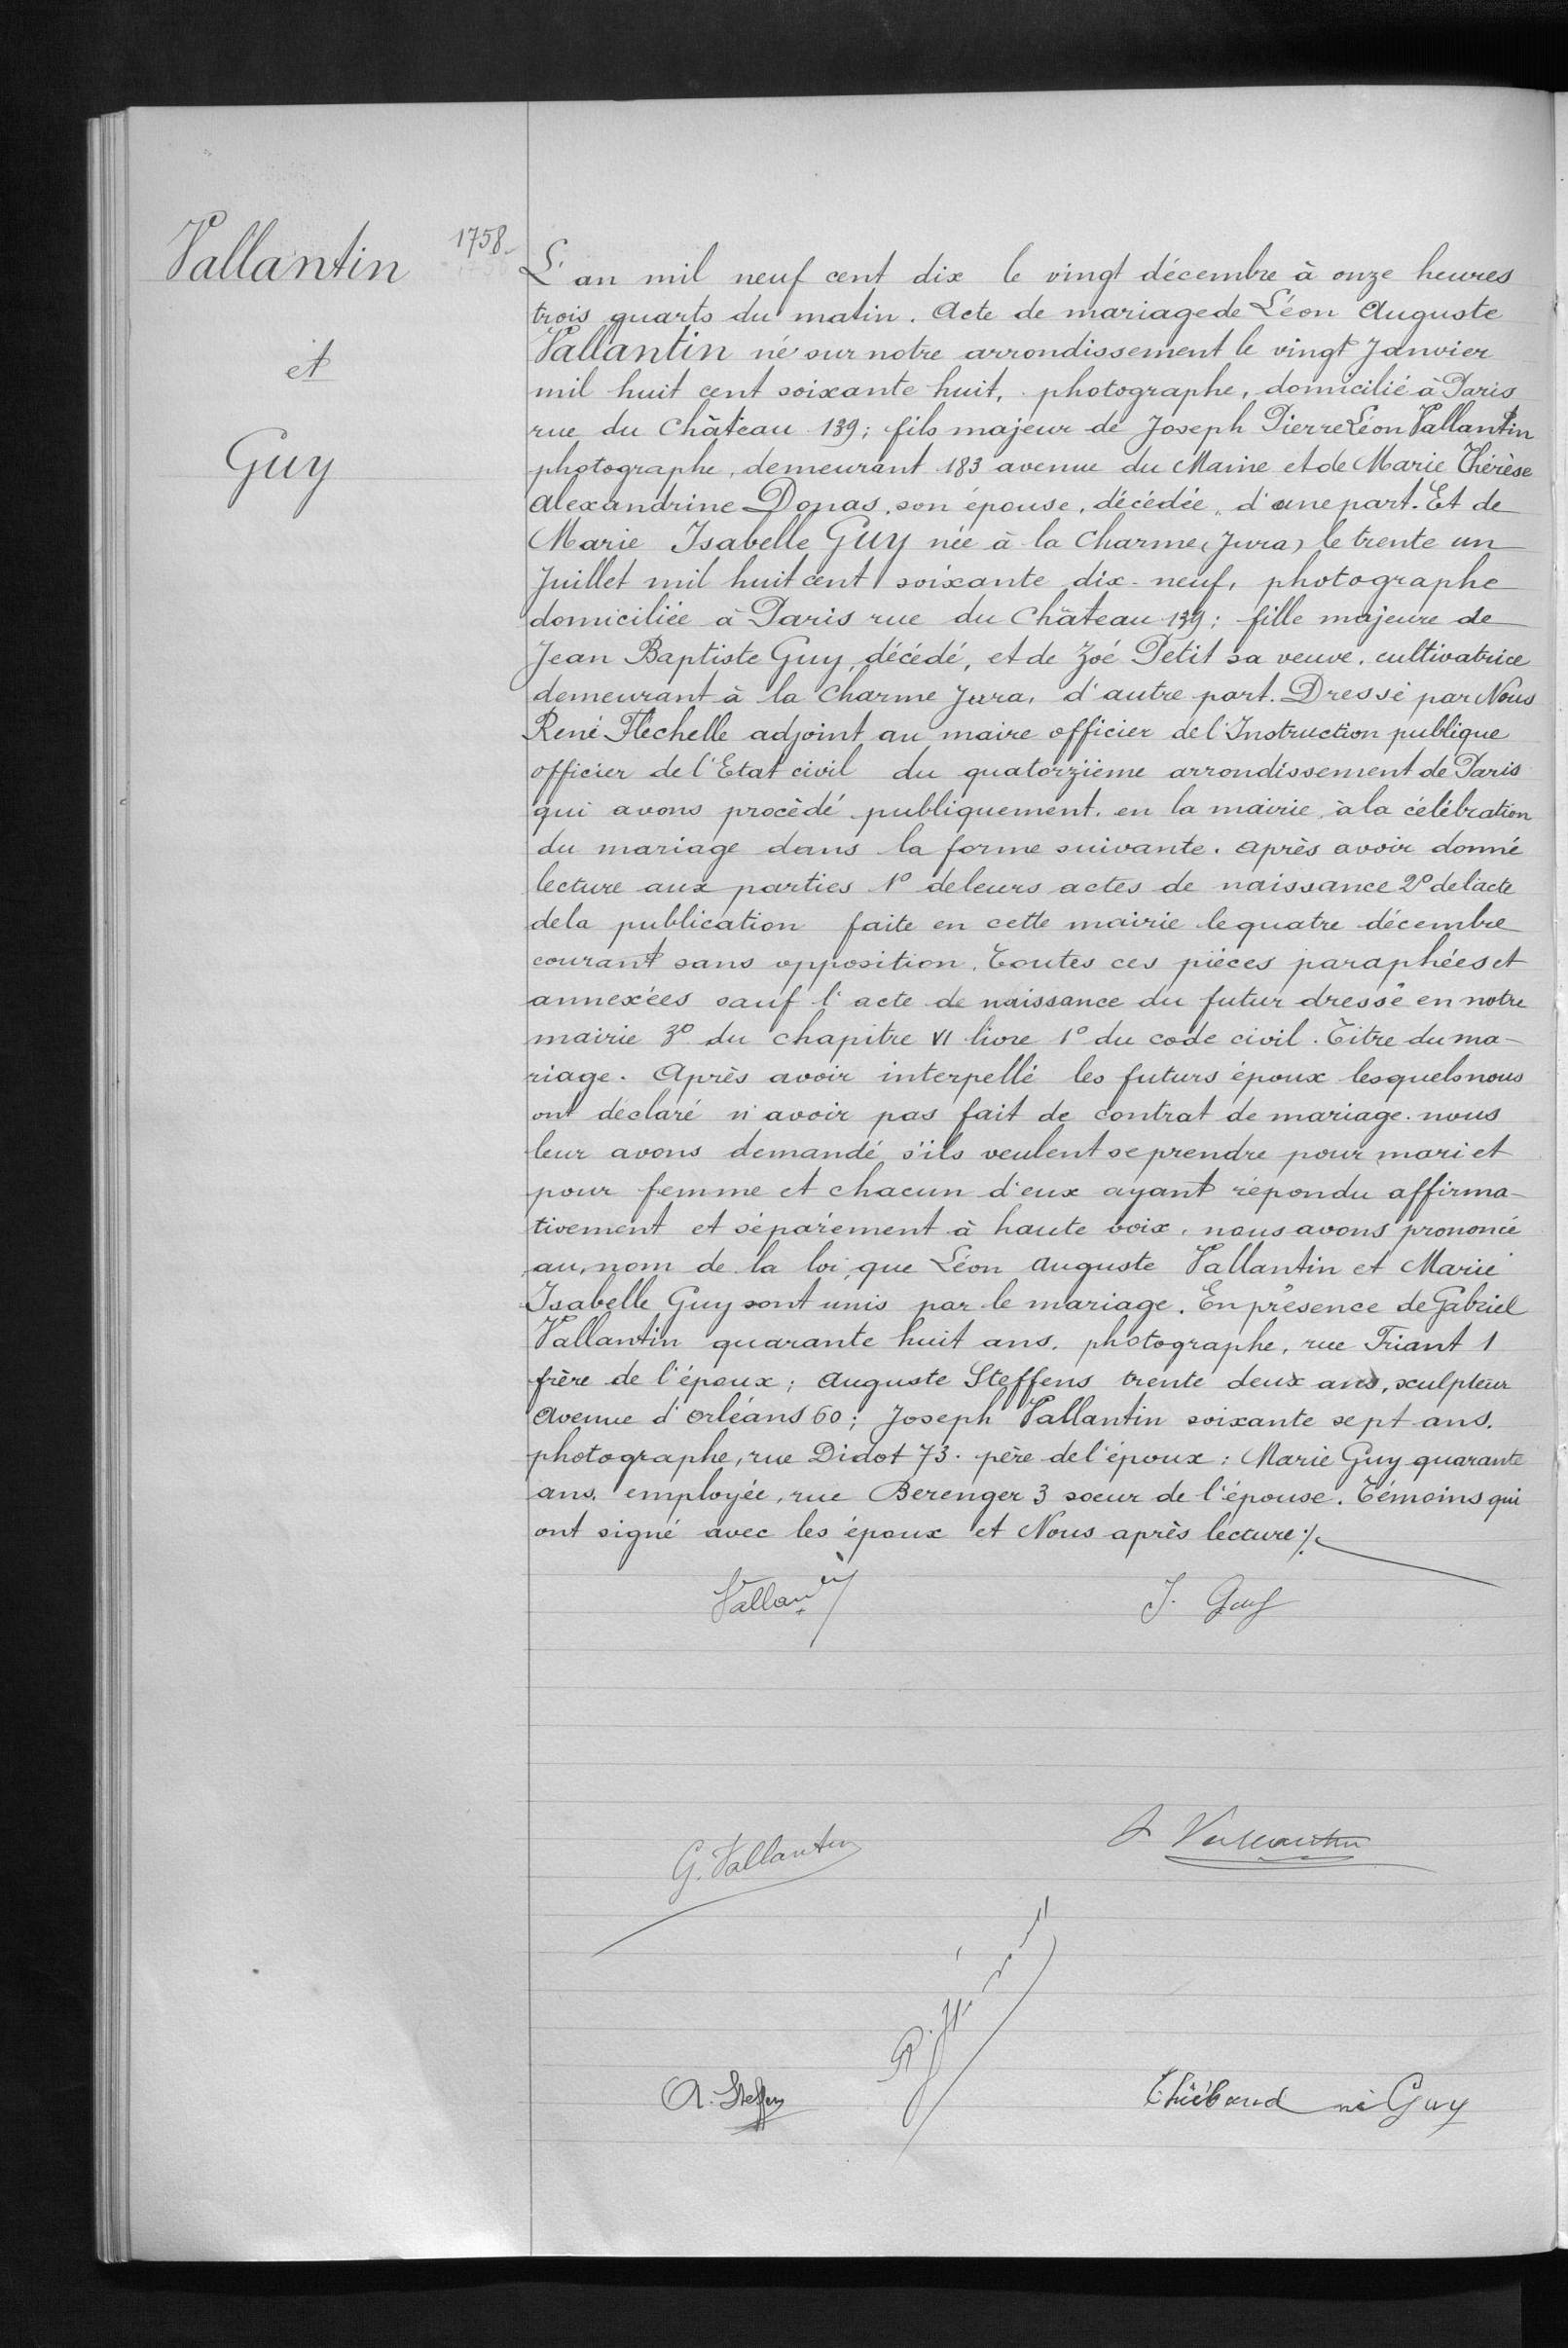1758
Vallantin
et
Guy
L'an mil neuf cent dix le vingt décembre à onze heures
trois quarts du matin. Acte de mariage de Léon Auguste
Vallantin né sur notre arrondissement le vingt Janvier
mil huit cent soixante huit, photographe, domicilié à Paris
rue du Château 139: fils majeur de Joseph Pierre Léon Vallantin
photographe, demeurant 183 avenue du Maire et de Marie Thérèse
Alexandrine Donas, son épouse, décédée, d'une part. Et de
Marie Isabelle Guy née à la Charme (Jura) le trente un
Juillet mil huit cent soixante dix-neuf, photographe
domiciliée à Paris rue du Château 139: fille majeure de
Jean Baptiste Guy, décédé, et de Zoé Petit sa veuve, cultivatrice
demeurant à la Charme Jura, d'autre part. Dressé par Nous
officier de l'Etat civil du quatorzième arrondissement de Paris
qui avons procédé publiquement en la mairie à la célébration
du mariage dans la forme suivante. après avoir donné
lecture aux parties 1° de leurs actes de naissance 2° de l'acte
de la publication faite en cette mairie le quatre décembre
courant sans opposition. Toutes ces pièces paraphées et
annexées sauf l'acte de naissance du futur dressé en notre
mairie 3° du chapitre VI livre 1° du code civil.; Titre du ma-
riage. Après avoir interpellé les futurs époux lesquels nous
ont déclaré n'avoir pas fait de contrat de mariage nous
leur avons demandé s'ils veulent se prendre pour mari et
pour femme et chacun d'eux ayant répondu affirma-
tivement et séparément à haute voix, nous avons prononcé
au nom de la loi que Léon Auguste Vallantin et Marie
Isabelle Guy sont unis par le mariage. En présence de Gabriel
Vallantin quarnte huit ans, photographe rue Friant 1
frère de l'époux: Auguste Steffens trente deux ans, sculpteur
Avenue d'Orléans 60; Joseph Vallantin soixante sept ans,
photographe, rue Didot 73, père de l'époux: Marie Guy quarante
ans, employée, rue Berenger 3 soeur de l'épouse, témoins qui
ont signé avec les époux et Nous après lecture./.
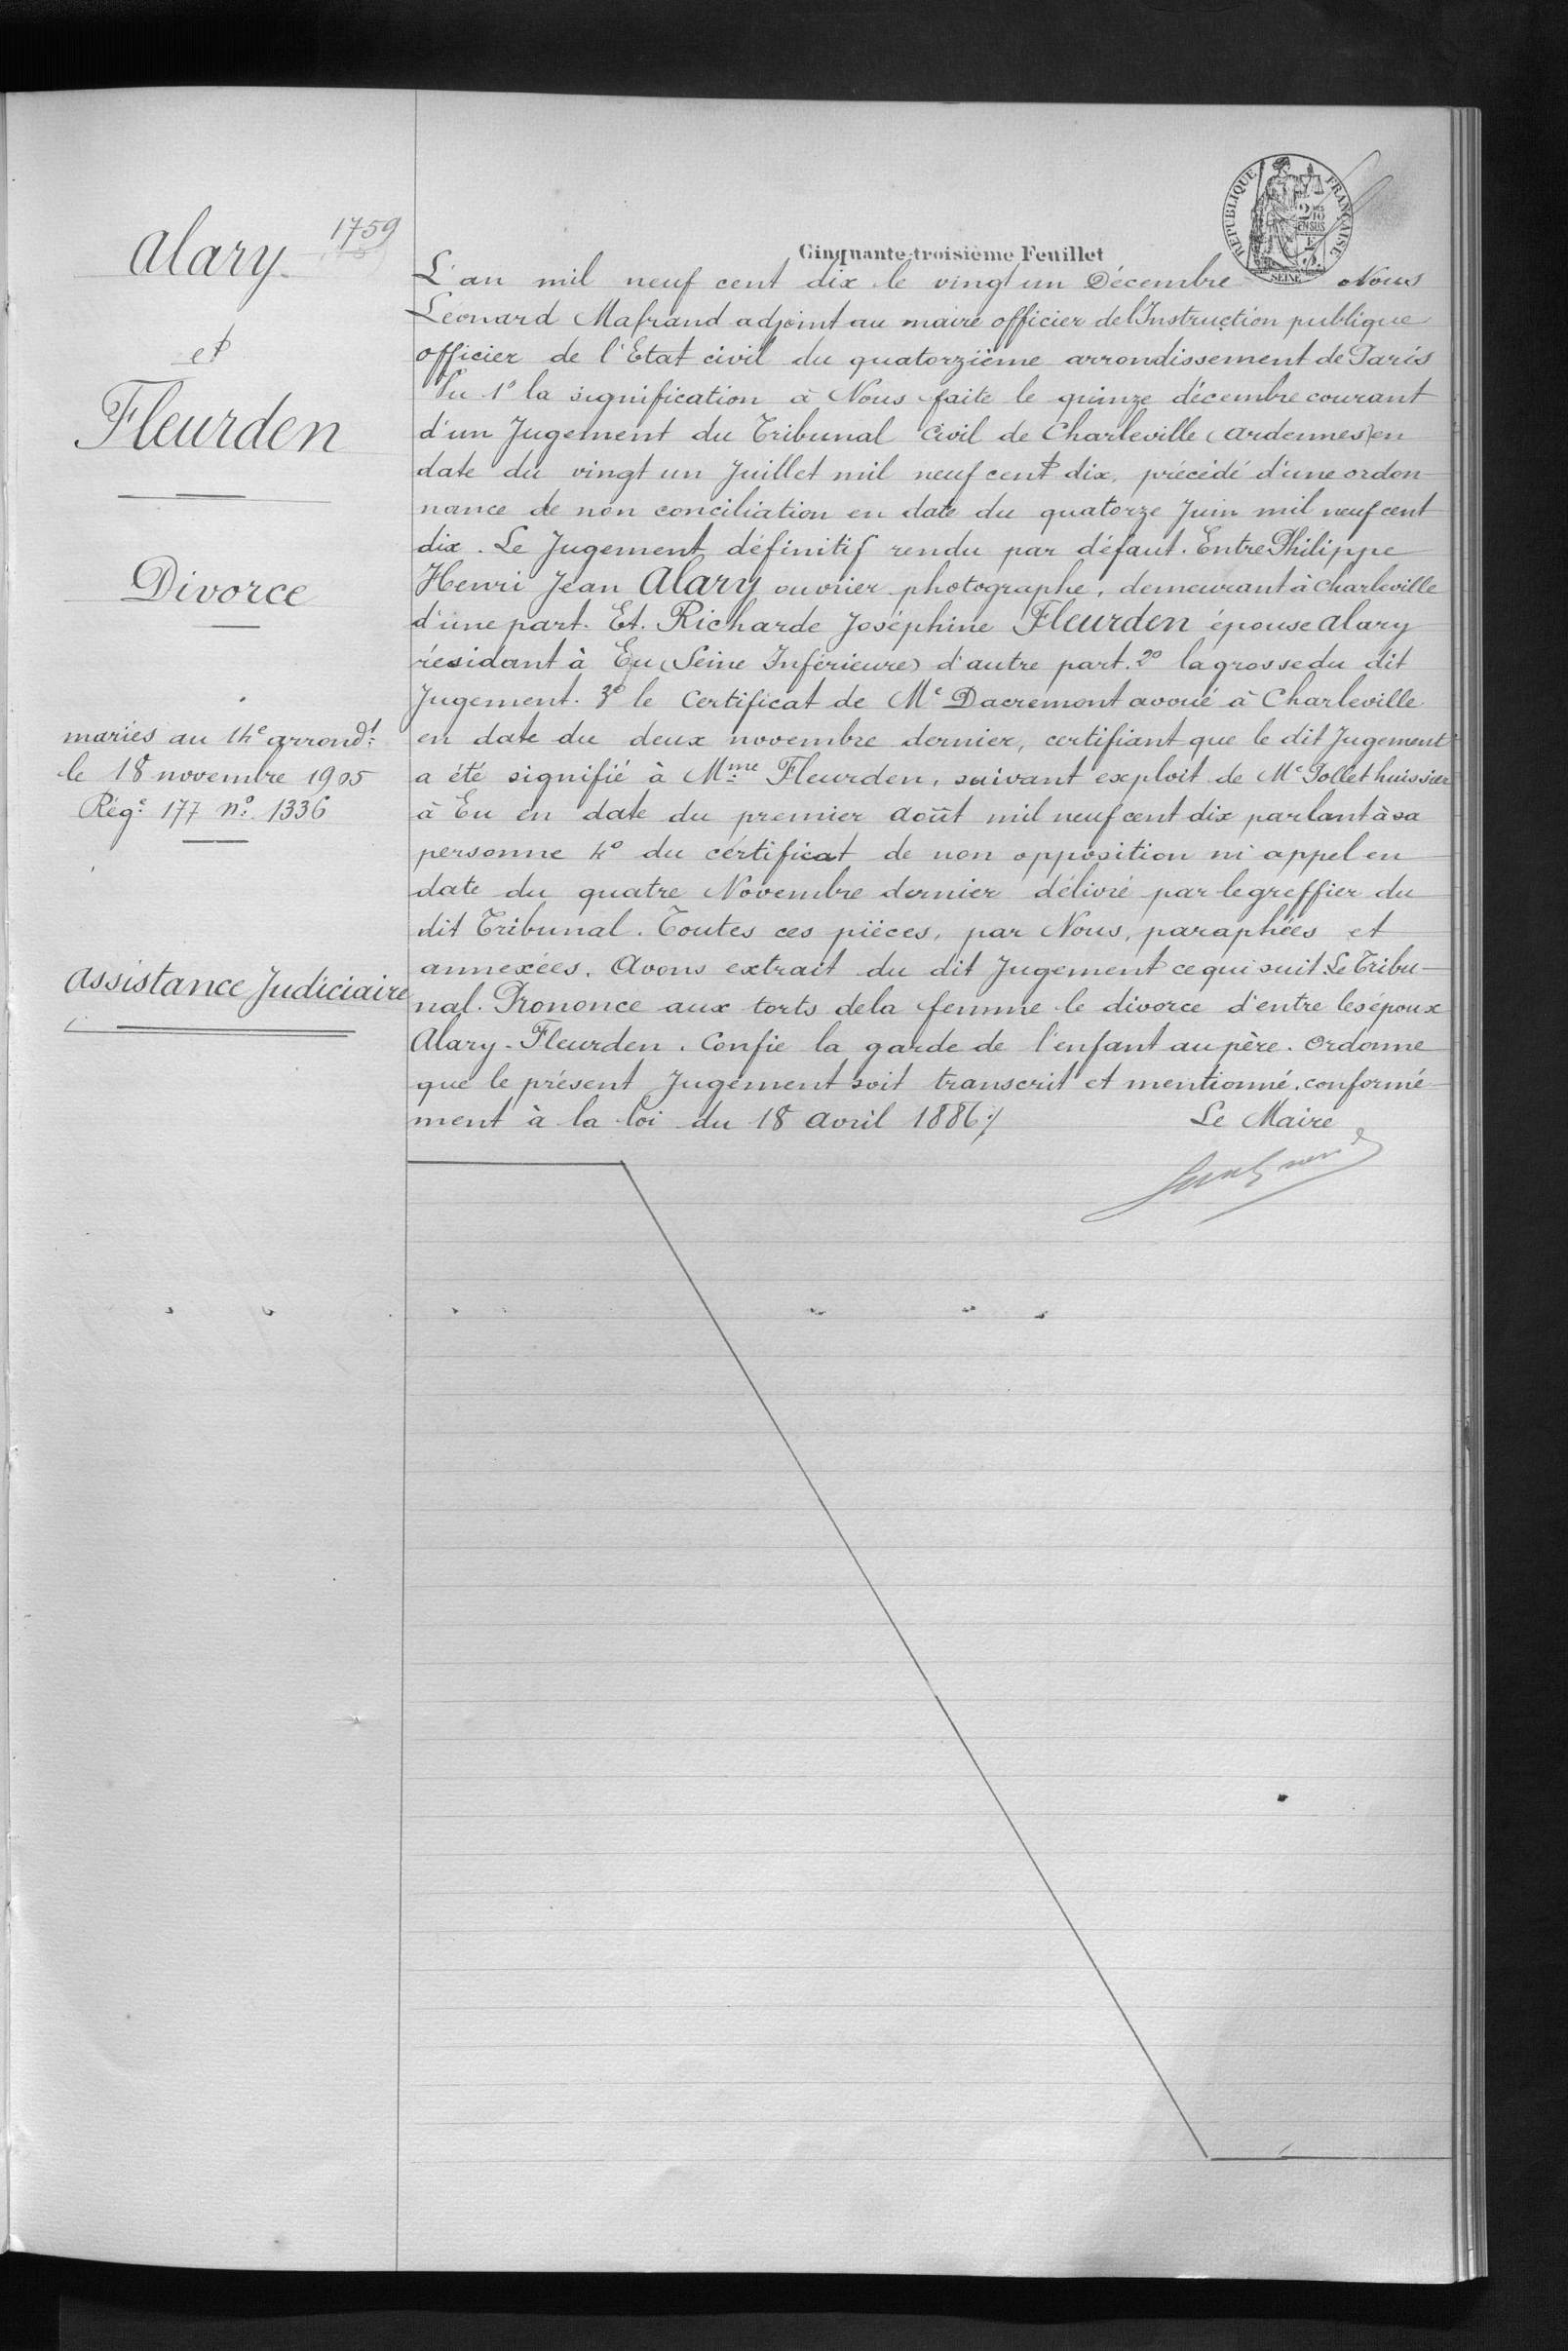1759
Alary
et
Fleurden
Divorce
L'an mil neuf cent dix le vingt un Décembre Nous
Léonard Mafrand adjoint au maire officier de l'Instruction publique
officier de l'Etat civil du quatorzième arrondissement de Paris
Vu 1° la signification à Nous faite le quinze décembre courant
d'un Jugement du Tribunal civil de Charleville (Ardennes) en
date du vingt un Juillet mil neuf cent dix, précédé d'une ordon-
nance de non conciliation en date du quatorze Juin mil neuf cent
dix. Le Jugement définitif rendu par défaut. Entre Philippe
Henri Jean Alary ouvrier photographe, demeurant à Charleville
d'une part. Et Richarde Joséphine Fleurden épouse Alary
résidant à Eu (Seine Inférieure) d'autre part. 2° la grosse du dit
Jugement. 3° le Certificat de Me Dacremont avoué à Charleville
en date du deux novembre dernier, certifiant que le dit Jugement
a été signifié à Mme Fleurden, suivant exploit de Me Pollet huissier
à Eu en date du premier août mil neuf cent dix parlant à sa
personne 4° du certificat de non opposition ni appel en
date du quatre Novembre dernier délivré par le greffier du
dit Tribunal. Toutes ces pièces, par Nous, paraphées et
annexées. Avons extrait du dit Jugement ce qui suit le Tribu-
nal. Prononce aux torts de la femme le divorce d'entre les époux
Alary-Fleurden, Confie la garde de l'enfant au père, ordonne
que le présent jugement soit transcrit et mentionné conformé
ment à la loi du 18 avril 1886 ./. Le Maire